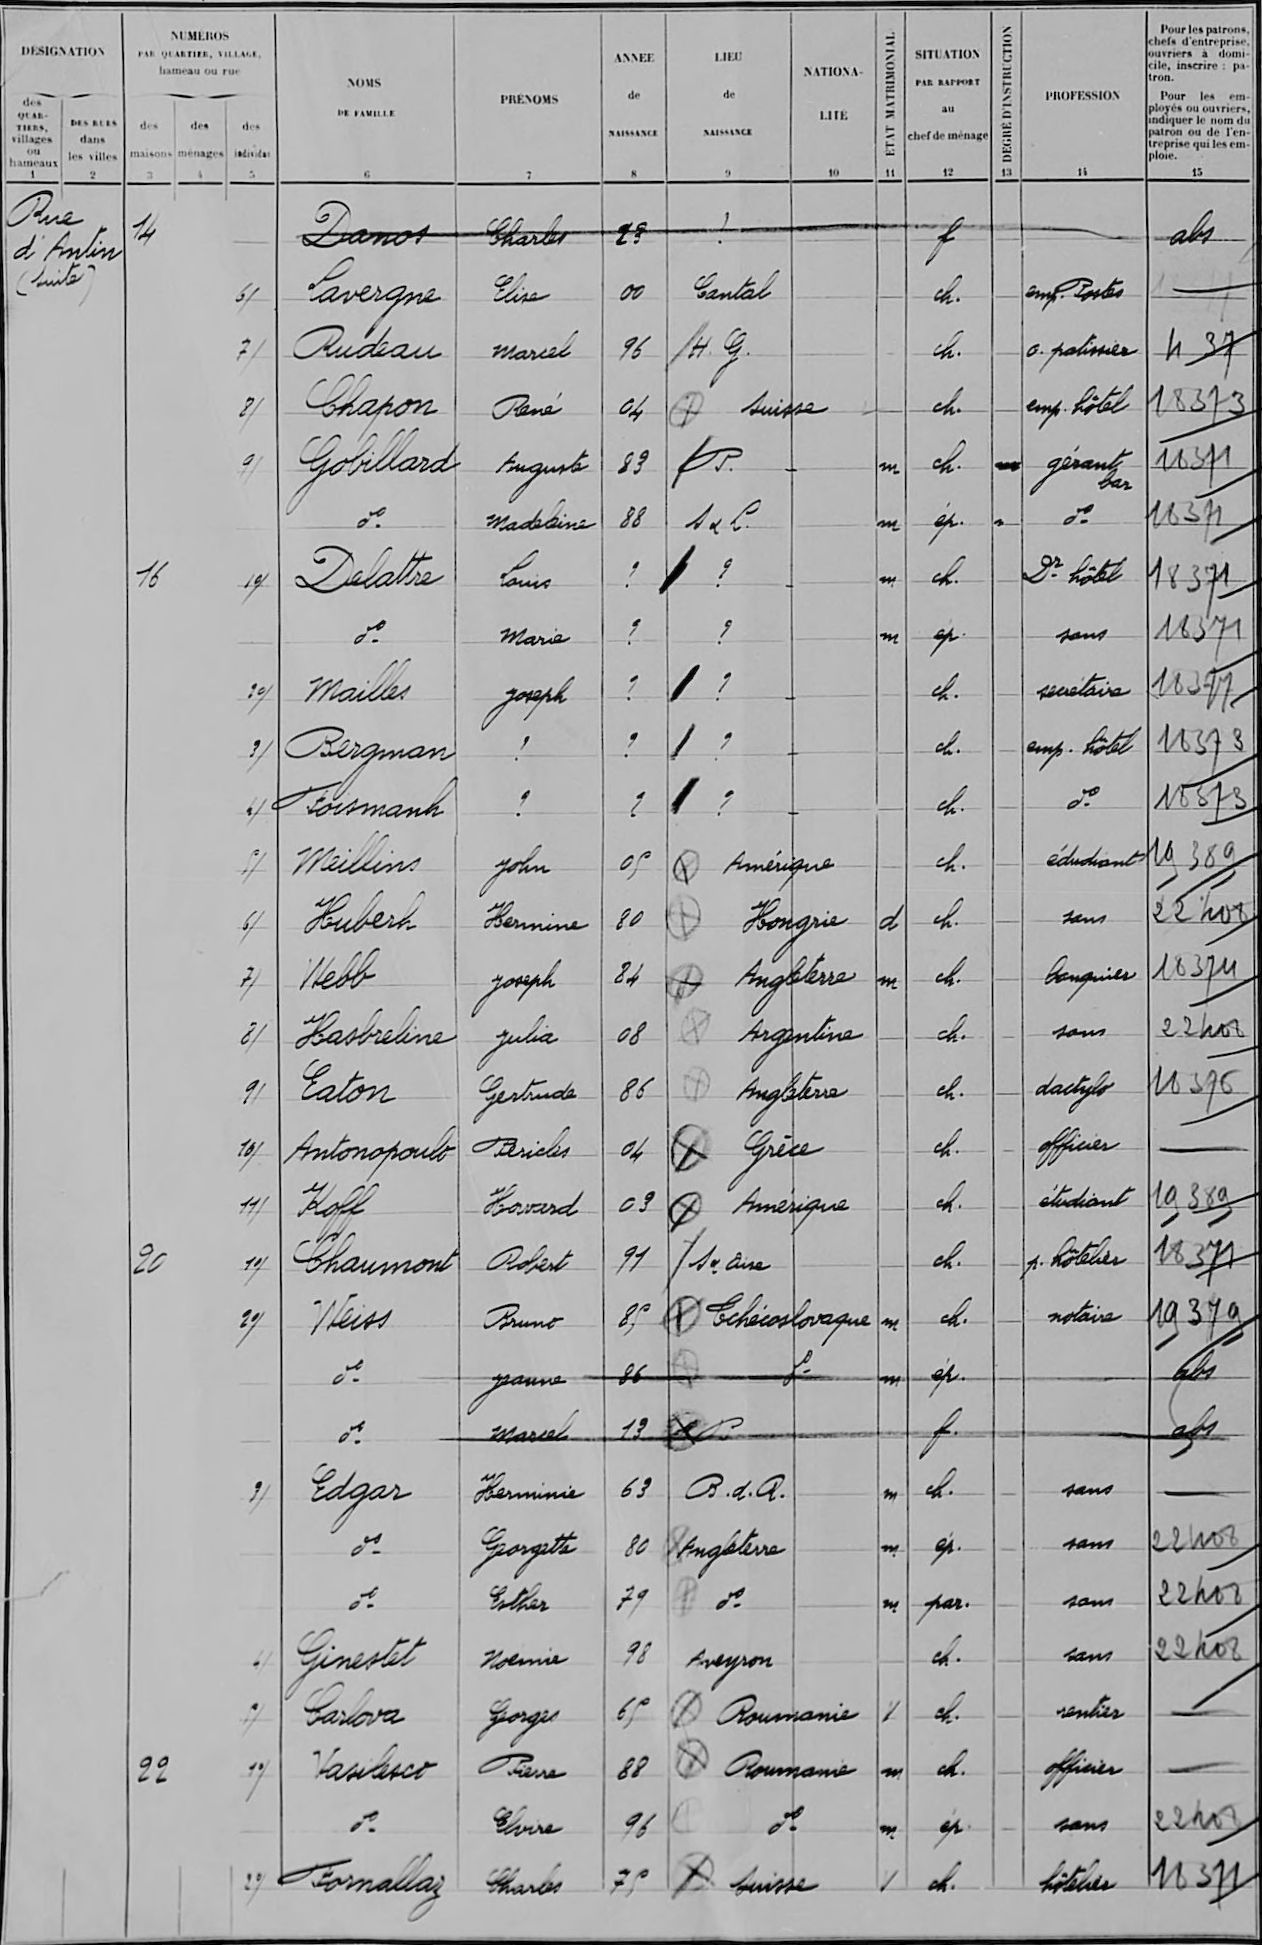Danot/Charles/23/¤/¤/¤/f/¤/¤/abs
Lavergne/Elise/00/Cantal/¤/¤/ch /¤/emp Postes/¤
Rudeau/marcel/96/H G /¤/¤/ch /¤/o patissier/437
Chapon/René/04/Suisse/¤/¤/ch /¤/emp hôtel/18373
Gobillard/Auguste/83/P /¤/m/ch /¤/gérant !bar/18371
d°/Madeleine/88/S & L/¤/m/ép /¤/d°/18371
Delattre/Louis/¤/¤/¤/m/ch /¤/Dr hôtel/18371
d°/Marie/¤/¤/¤/m/ep/¤/sans/18371
Mailles/joseph/¤/¤/¤/¤/ch /¤/secrétaire/18377
Bergman/¤/¤/¤/¤/¤/ch /¤/emp hôtel/18373
Foismanh/¤/¤/¤/¤/¤/ch /¤/d°/18373
Meillins/john/05/Amérique/¤/¤/ch /¤/étudiant/19389
Huberh/Hermine/80/Hongrie/¤/d/ch /¤/sans/22408
Webb/joseph/84/Angleterre/¤/m/ch /¤/banquier/18374
Hasbreline/julia/08/Argentine/¤/¤/ch /¤/sans/22408
Eaton/Gertrude/86/Angleterre/¤/¤/ch /¤/dactylo/18376
Antonopoulo/Pericles/04/Grèce/¤/¤/ch /¤/officier/¤
Koff/Howard/03/Amérique/¤/¤/ch /¤/étudiant/19389
Chaumont/Robert/91/S et Oise/¤/¤/ch /¤/p hôtelier/18371
Weiss/Bruno/85/Tchécoslovaque/¤/m/ch /¤/notaire/19379
d°/jeanne/86/d°/¤/m/ep/¤/¤/abs
d°/marcel/13/P/¤/¤/f /¤/¤/abs
Edgar/Herminie/63/B d R /¤/m/ép /¤/sans/¤
d°/Georgette/80/Angleterre/¤/m/ép /¤/sans/22408
d°/Esther/79/d°/¤/m/par /¤/sans/22408
Ginestet/Noémie/98/Aveyron/¤/¤/ch /¤/sans/22408
Carlova/Georges/65/Roumanie/¤/V/ch /¤/rentier/¤
Vasilesco/Pierre/88/Roumanie/¤/m/ch /¤/officier/¤
d°/Elvire/96/d°/¤/m/ép /¤/sans/22408
Fornallaz/Charles/75/Suisse/¤/V/ch /¤/hôtelier/18371
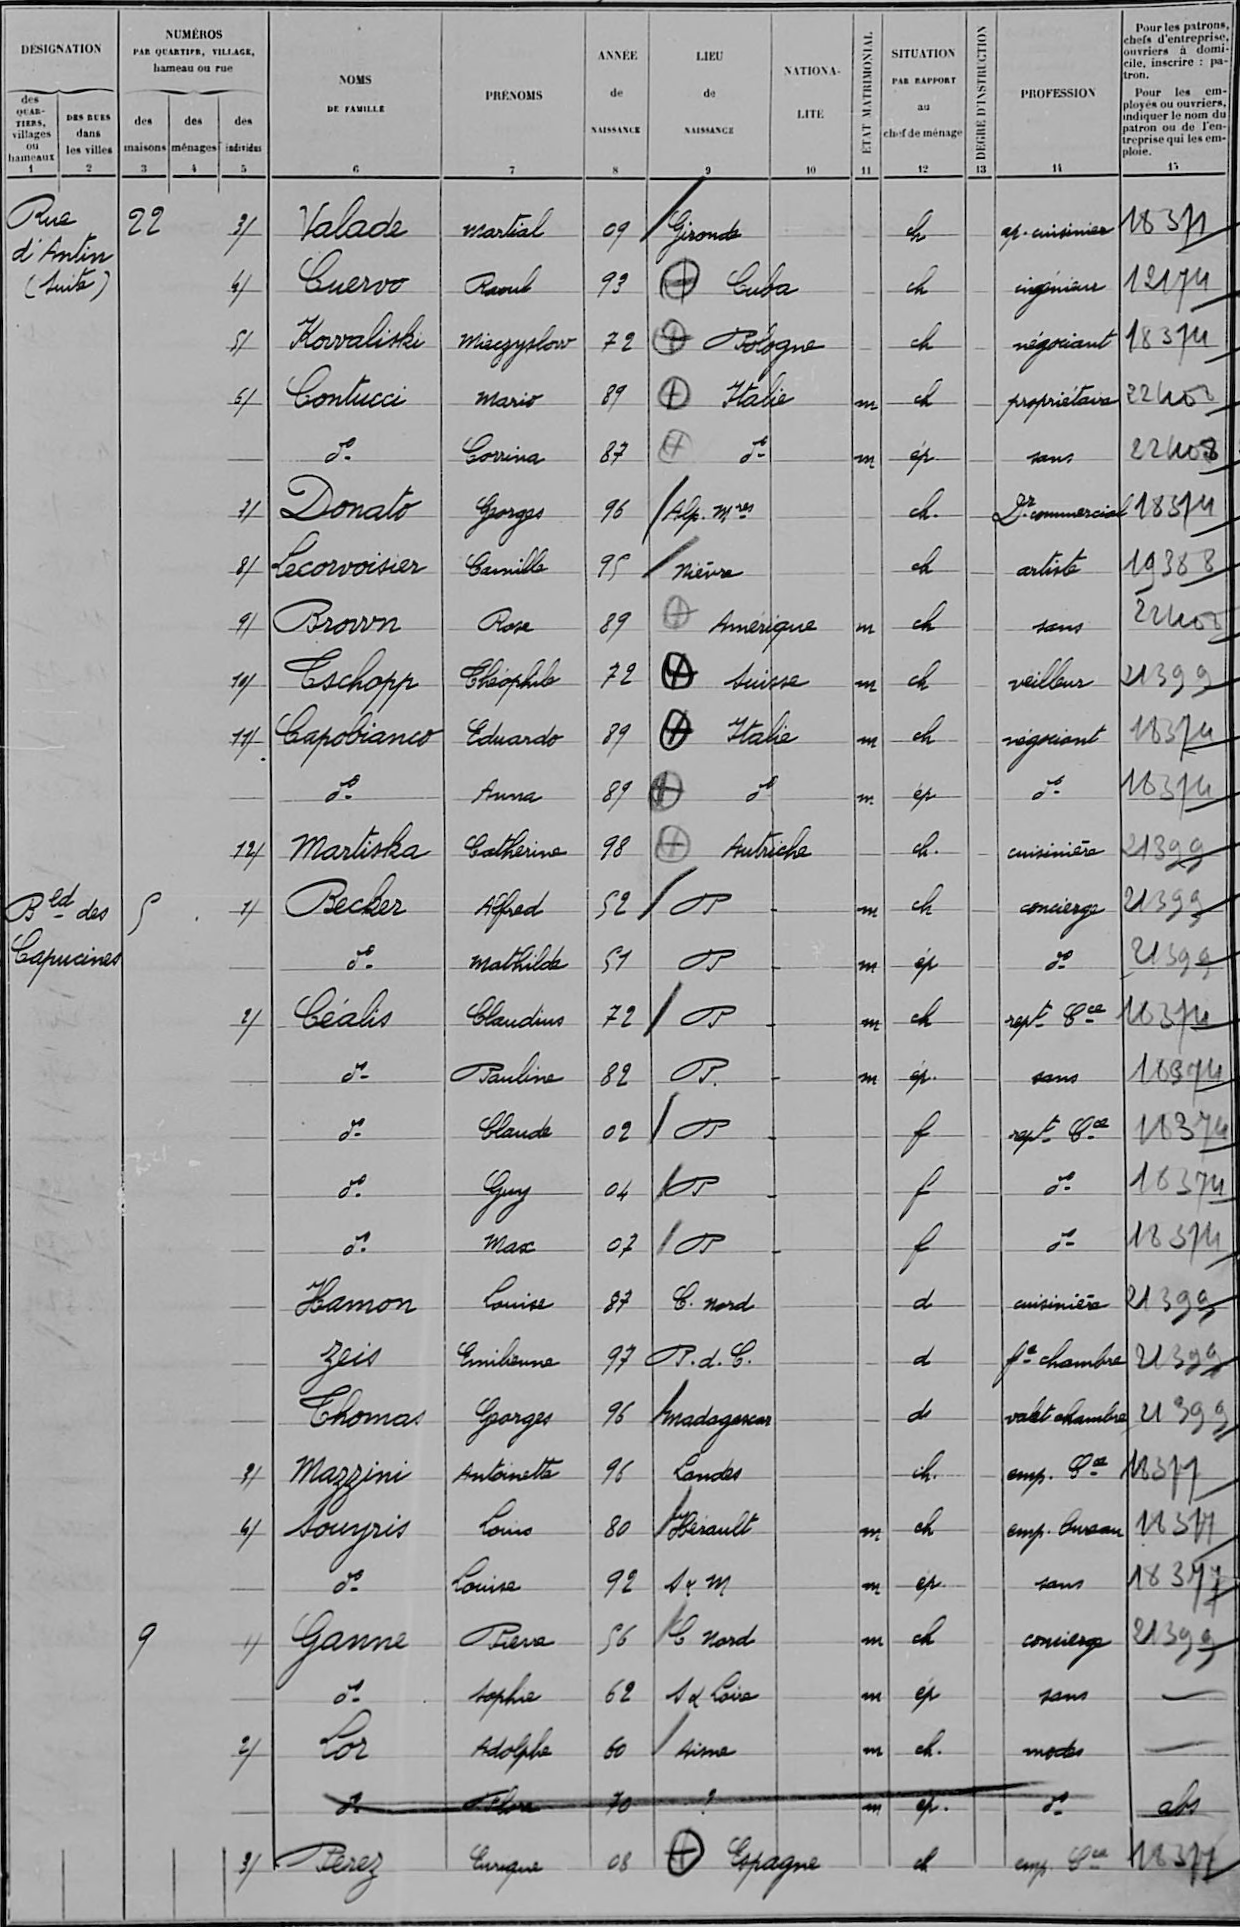Valade/martial/09/Gironde/¤/¤/ch/¤/ap cuisinier/18371
Cuervo/Raoul/93/Cuba/¤/¤/ch/¤/ingénieur/12174
Korvaliski/Mieczyslow/72/Pologne/¤/¤/ch/¤/négociant/18374
Contucci/Mario/89/Italie/¤/m/ch/¤/propriétaire/22408
d°/Corrina/87/d°/¤/m/ép /¤/sans/22408
Donato/Georges/96/Alp Mes/¤/¤/ch /¤/Dr commercial/18374
Lecorvoisier/Camille/95/Nièvre/¤/¤/ch/¤/artiste/19388
Brown/Rose/89/Amérique/¤/m/ch/¤/sans/22408
Tschopp/Théophile/72/Suisse/¤/m/ch/¤/veilleur/21399
Capobianco/Eduardo/89/Italie/¤/m/ch/¤/négociant/18374
d°/Anna/89/d°/¤/m/ép/¤/d°/18374
Martiska/Catherine/98/Autriche/¤/¤/ch /¤/cuisinière/21399
Becker/Alfred/52/P/¤/m/ch/¤/concierge/21399
d°/mathilde/51/P/¤/m/ép/¤/d°/21399
Céalis/Claudius/72/P/¤/m/ch/¤/rept Cce/18374
d°/Pauline/82/P/¤/m/ép/¤/sans/18374
d°/Claude/02/P/¤/¤/f/¤/rept Cce/18374
d°/Guy/04/P/¤/¤/f/¤/d°/18374
d°/Max/07/P/¤/¤/f/¤/d°/18374
Hamon/Louise/87/C Nord/¤/¤/d/¤/cuisinière/21399
Zeis/Emilienne/97/P d C /¤/¤/d/¤/fe chambre/21399
Thomas/Georges/96/Madagascar/¤/¤/d/¤/valet chambre/21399
Mazzini/Antoinette/96/Landes/¤/¤/ch/¤/emp Cce/18377
Souyris/Louis/80/Hérault/¤/m/ch/¤/emp bureau/18377
d°/Louise/92/S & M/¤/m/ép/¤/sans/18377
Ganne/Pierre/56/C Nord/¤/m/ch/¤/concierge/21399
d°/Sophie/62/S & Loire/¤/m/ép/¤/sans/¤
Lor/Adolphe/60/Aisne/¤/m/ch /¤/modes/¤
d°/Flore/70/¤/¤/m/ep /¤/d°/abs
Perez/Enrique/08/Espagne/¤/¤/ch/¤/emp Cce/18377